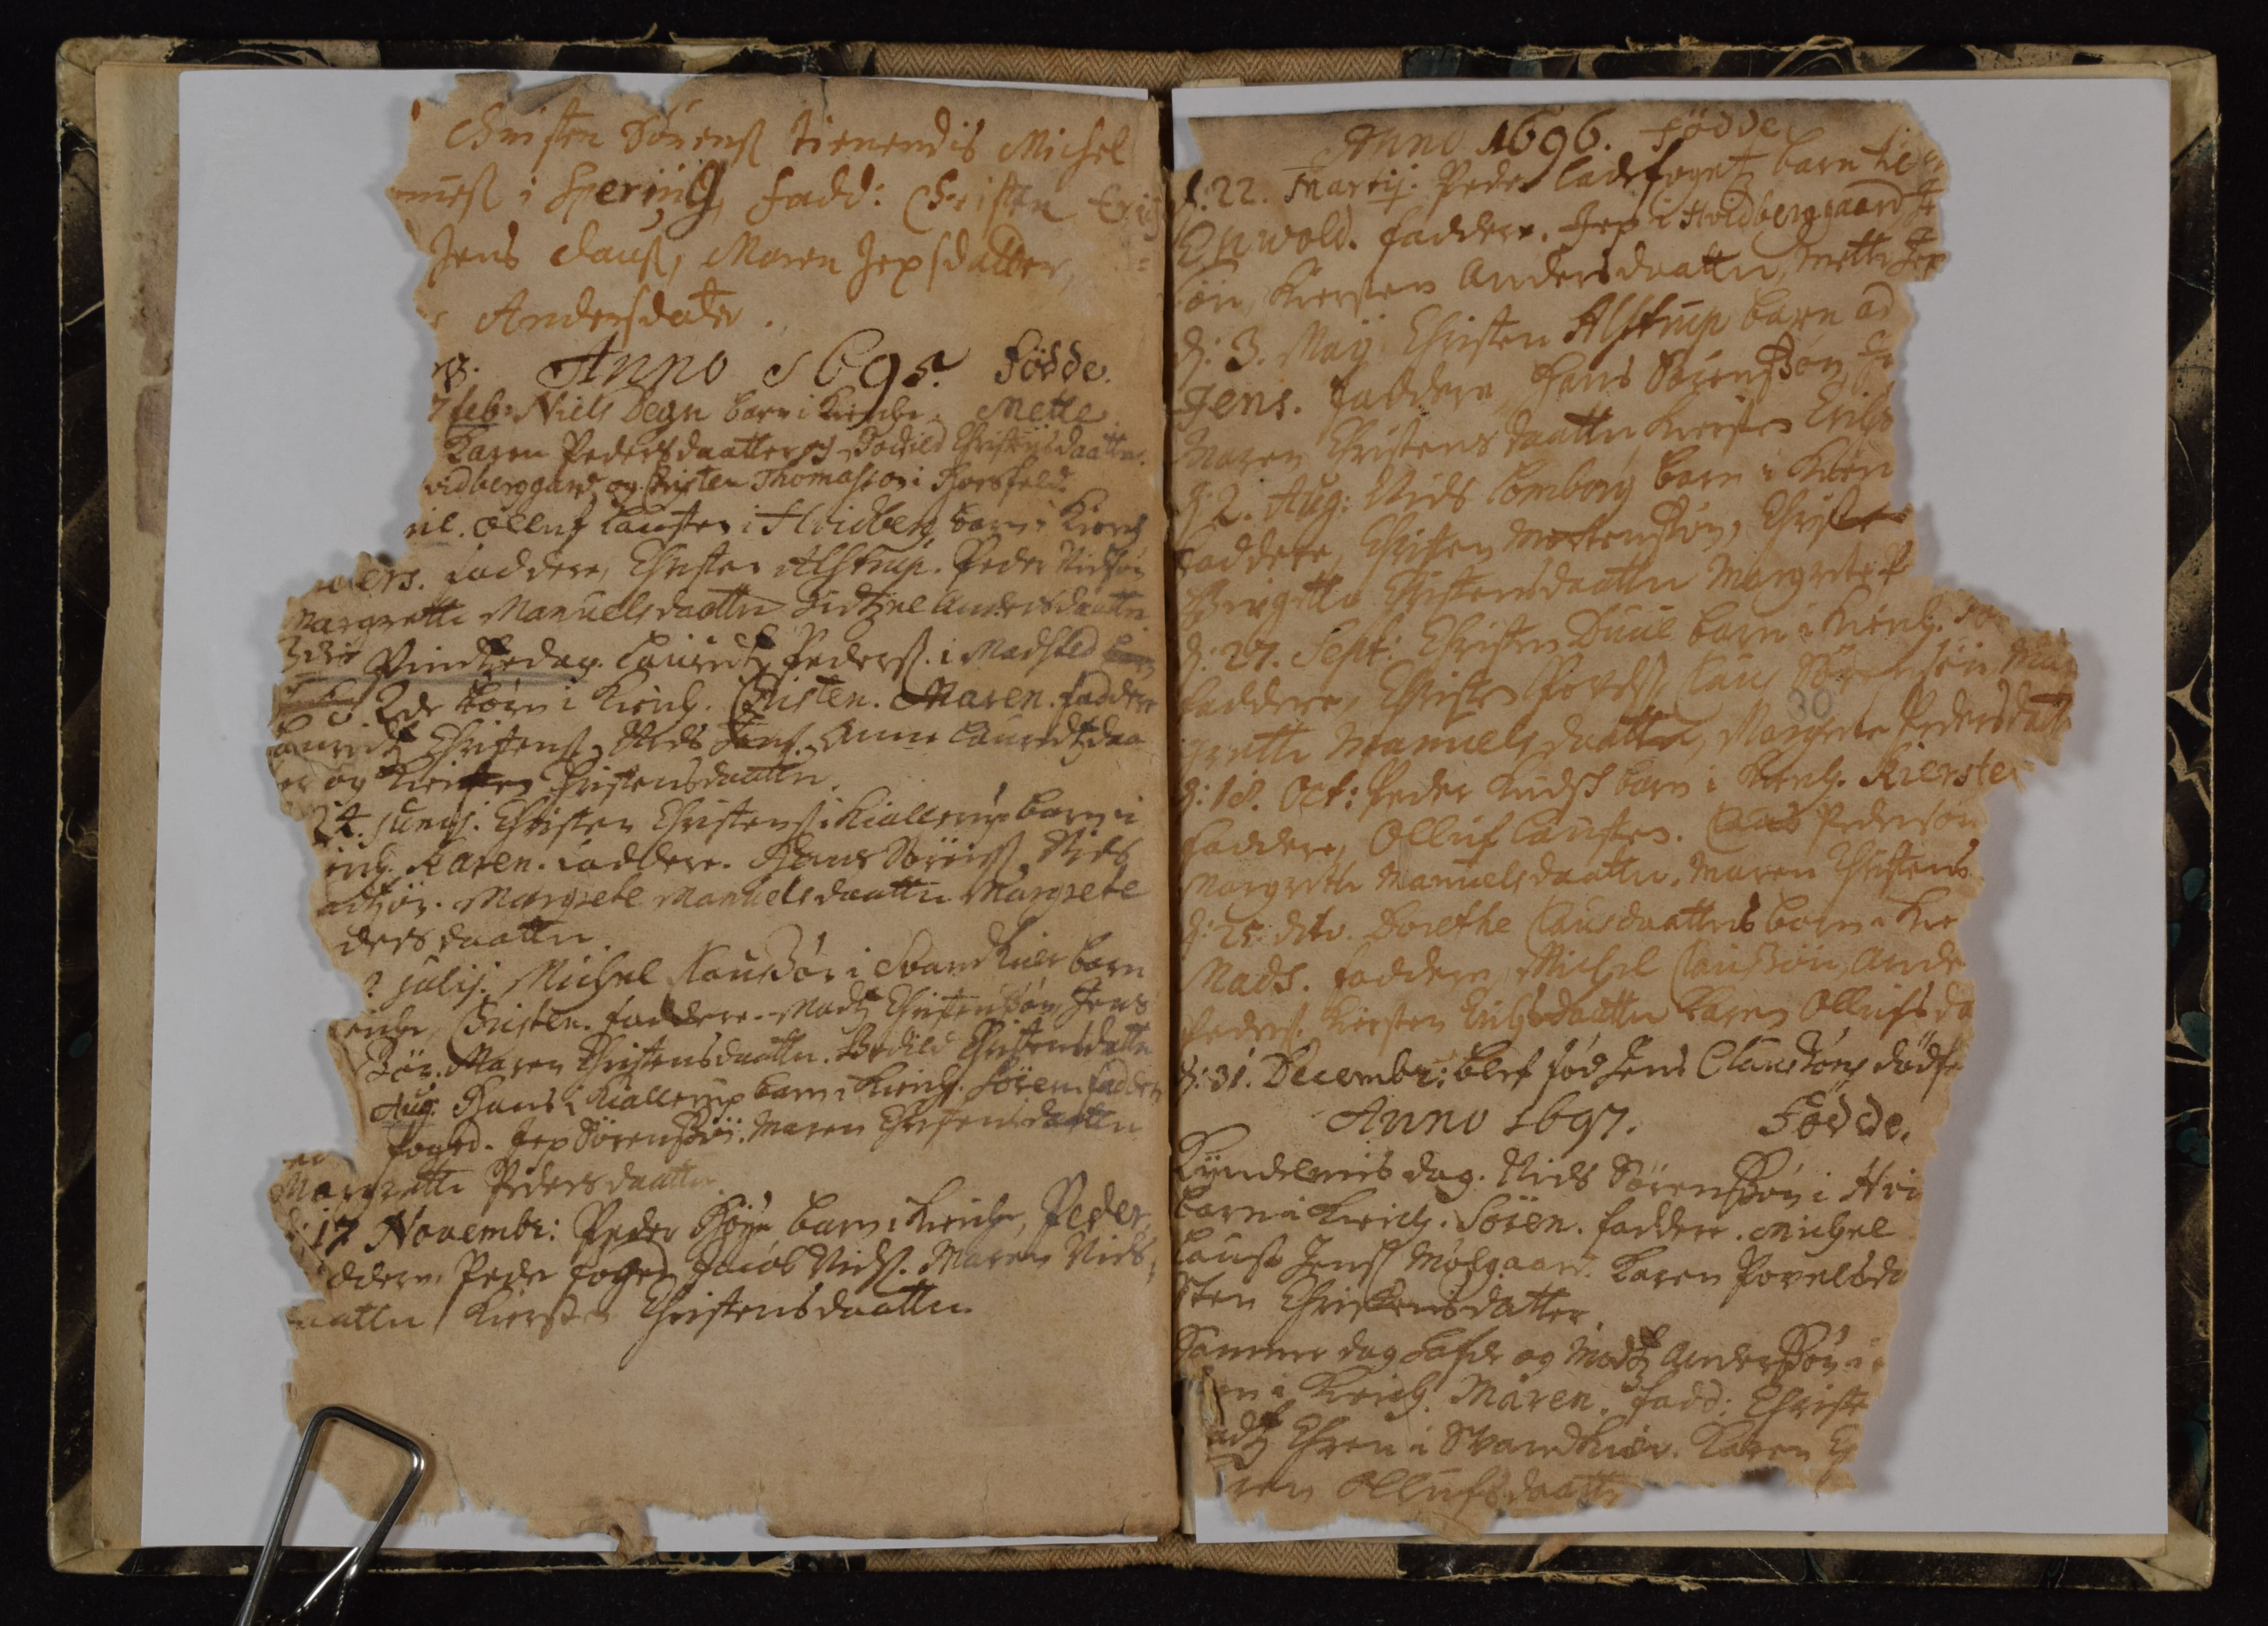Cirsten Sørens tienendis Michel
ust i Spering, Fadd: Christen Eri
Jens claus, Maren Jepsdatter
Andersdatter.
Anno 1695 Fødde
7 Feb: Niels Degn barn i Kirche Mette
Karen Pedersdaatter ## Bodild christensdaater
vidberggard og chriten Thomasøn Tjorsiheld
## Olluf Lausten i Hvidberg, barn i Kierch,
ders. Faddere, Christen Alstrup. Peder Nielsøn
Margrette Manuelsdaatter Zidtzel Andersdaatter
3die Pindtzedag Lauridtz Peders i Madsted ##
## 2de børn i Kirch Christen. Maren. Faddere
Lauridtz Christens Niels Jens Anne Lauridtzdaa
er og Kierten Christensdaatter.
24. Junij. Christen Christens i Kiallerup barn i
rche Karen. Faddere. Hans Sørens Niles
ritzøn. Margrete Manuelsdaatter Margrete
dersdaatter
2 Julij. Michel Claussøn i Svandkier barn
che, Christen Faddere. Madtz Christenssøn, Jens
##øn. Maren Christensdaatter. Bodild Christensdatter
Aug: Hans i Kiallerup barn i Kirche Søren Fadder
Foged. Jep Sørenssøn. Maren Christensdaater
Margrette Pedersdaatter
17 Nouembr: Peder Køye barn i Kirche, Peder
ddere. Peder Foged Jacob Niels. Maren Niels
aatter Kiersten Christensdaatter
Anno 1696.
Fødde
d. 22. Martij: Pedr Ladefoget barn til
Enwold. Faddere. Jep i Hvidberggaard Je
søn, Kiersten Andersdaatter, Mette Jep
d: 3. Maij Christen Alstrup barn ad  
Jens. Faddere Hans Sørenssøn, ##
Maren Christensdaatter Kiersten E##
D 2: Aug: Niels Lomborg barn i Kirc
Faddere, Christen Mortensøn, Christen
Birgete Christensdaatter Mergrete Pe
d: 27: Sept: Christen Duul barn i Kirche##
Faddere, Christen Povels ClausSørens Ni##
grette Manuels daatter, Margrete Pedersda
d: 18 Oct: Peder Kudsk barn i Kirce. Kierste
Faddere Olluf Laustens Claus Pedersøn
Margrethe Manuelsdaatter. Maren Christens
d: 25. oct: Dorethe Clausdaatters baren i Kir
Mads. Faddere Michel Clausøn Ande
Peders Kirsten ErichsdatterKaren Ollufsda
D: 31: Decembr: blef fød Jens Claussøns dødfø
Anno 1607
Fødde.
Kyndelmis dag. Niels Sørenssøn i Hvi
barn i Kirche. Søren. Faddere Michel
Laust Jens Mølgaard. Karen Povelda
sten Christensdatter.
Samme dag hafde og Madtz Anderssøn i
rn i Kirch. Maren. Fadd: Christe
adtz Chren i Svandkiær. Karen E
##en ollufsdaatter
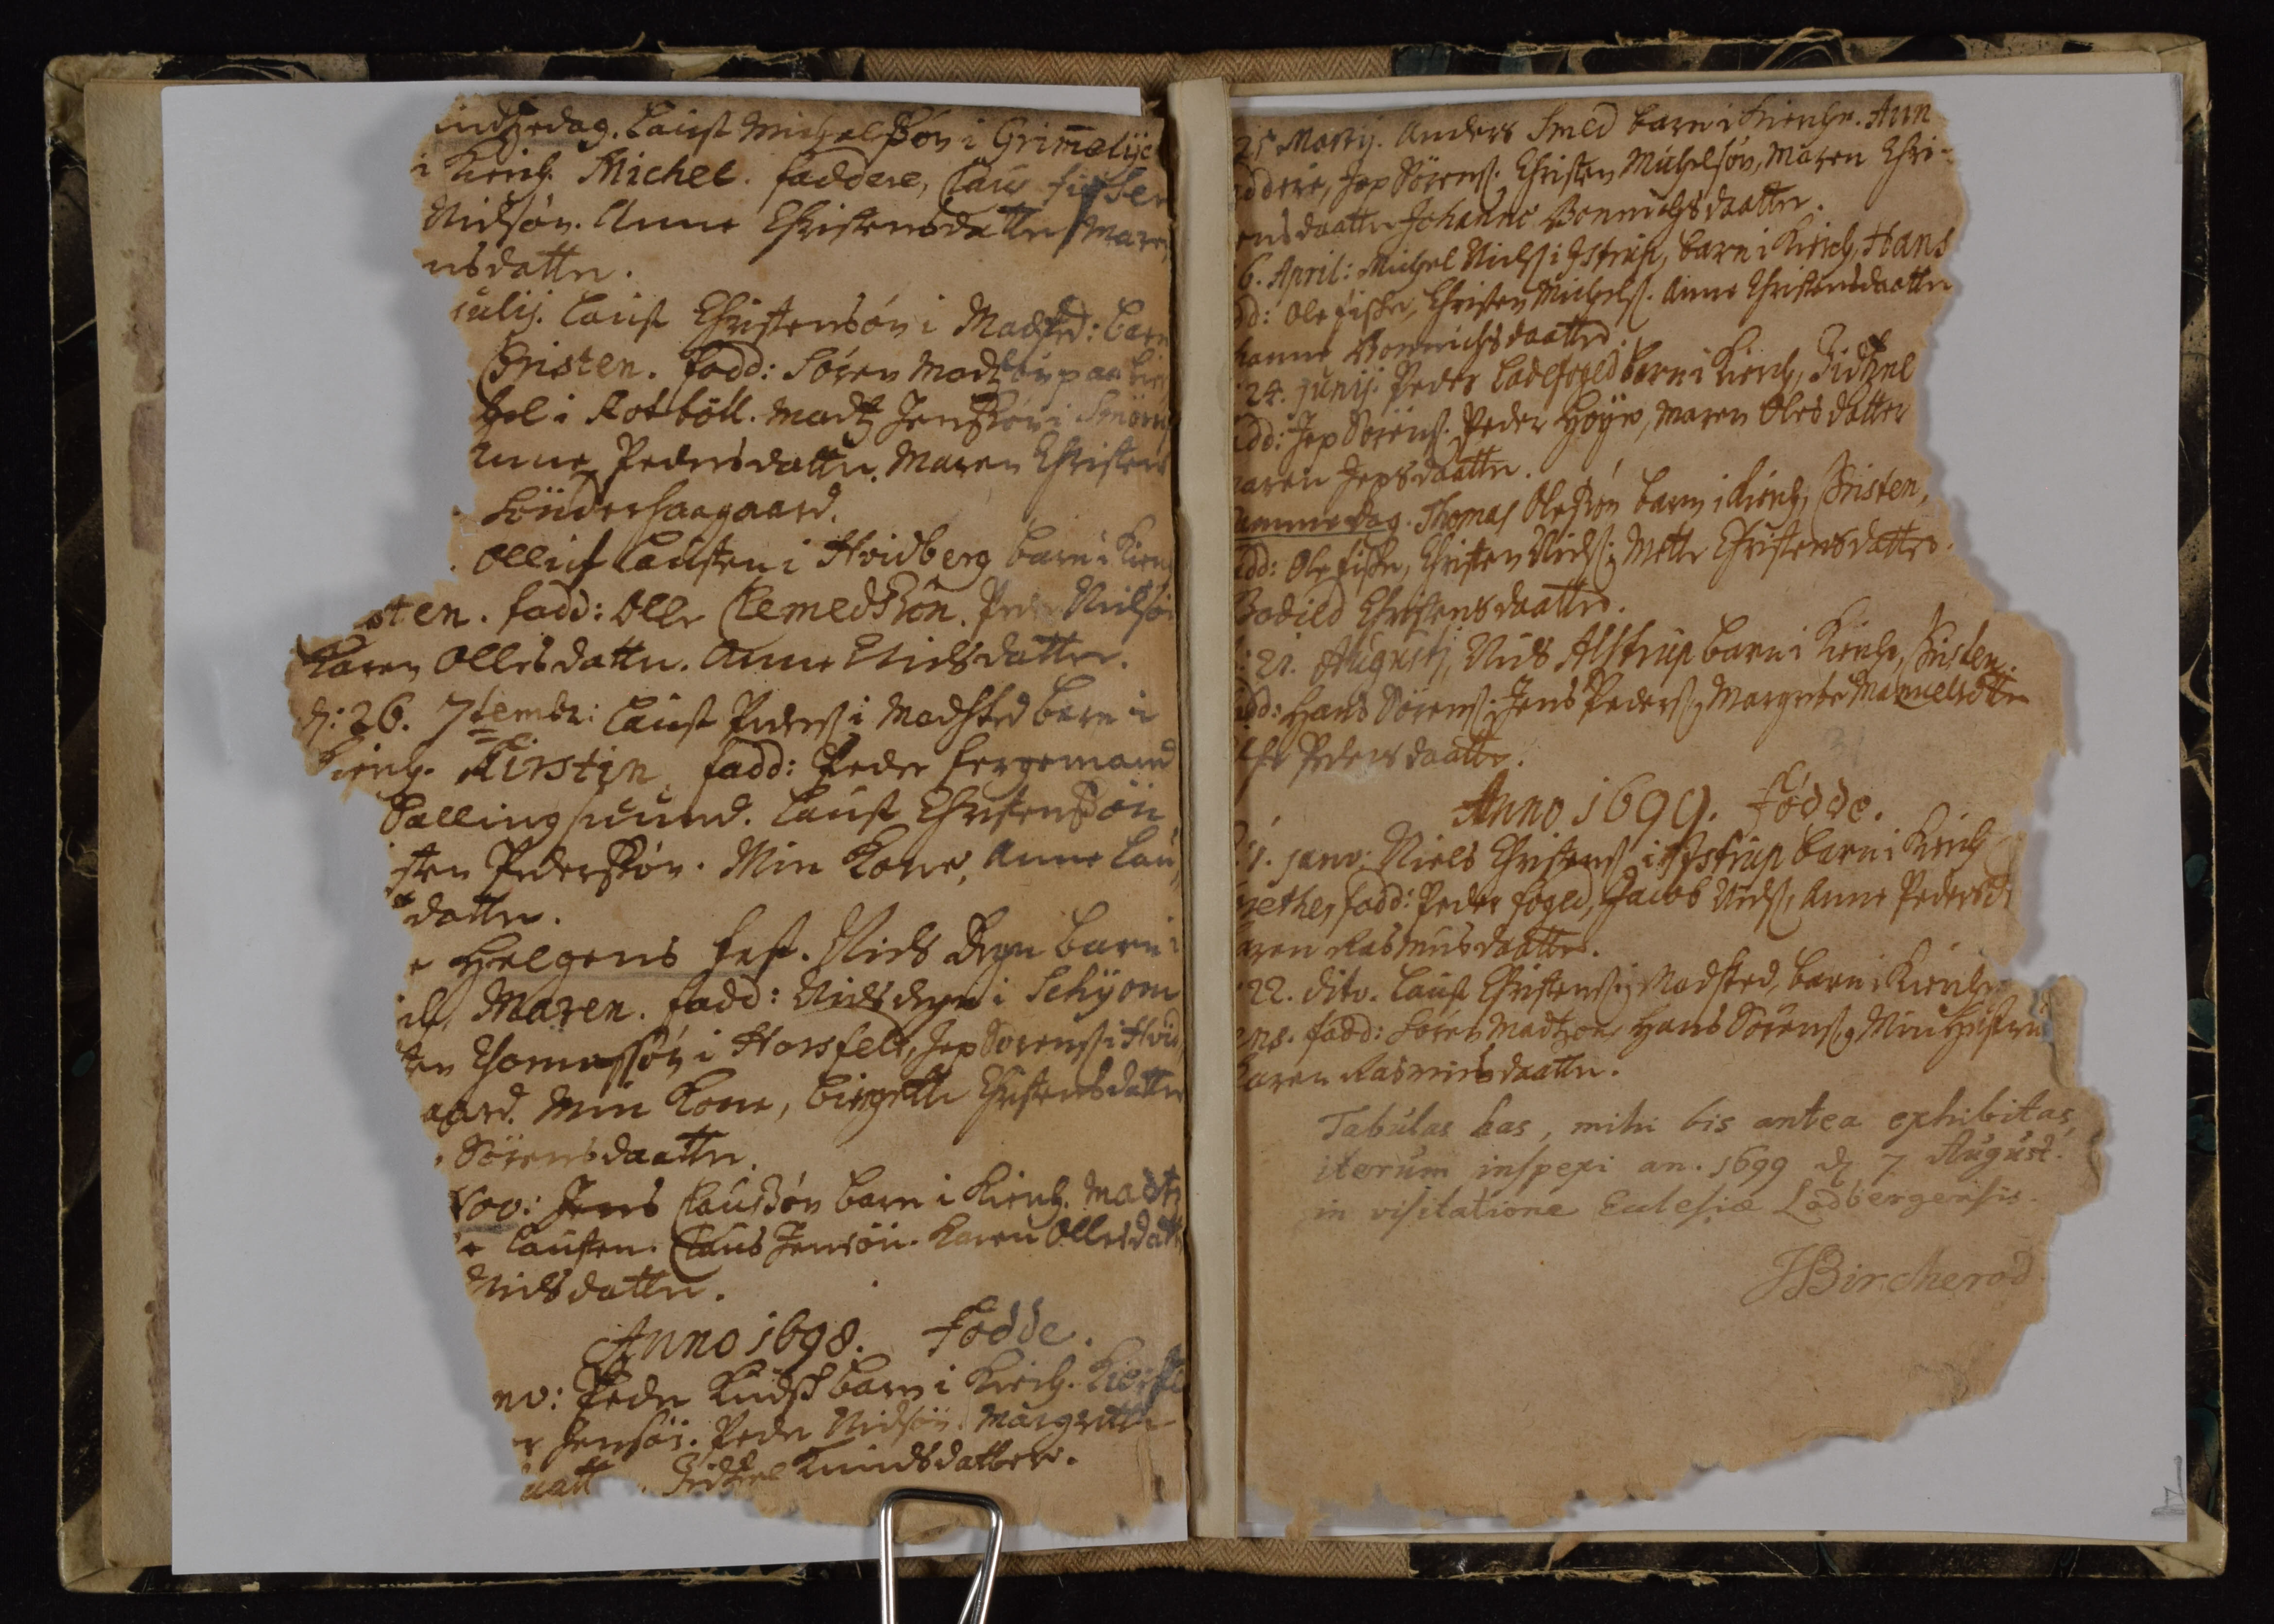indtzedag. Hans Michelssøn i Grimmolyc
i Kirch Michel. Faddere, Caus Fisher
Nielsøn. Anne Christensdatter Maren
nsdatter.
Julij. Laust Christensøn i Madsted: barn
Christen. Fadd: Søren Madtzøn paa ##
bol i Rotbøll. Madtz Jensssøn i Sønder##
Anne Pedersdatter. Maren Christens
Sønderhssgaard.
Olluf Lausten i Hvidberg barn Kir
ten. fadd: Olle Clemedssøn. Peder Nielsøn
Karen Ollesdater. Anne Nielsdatter.
d: 26. 7tembr. Laust Peders i Madsted barn i
Kirche Kirstin, fadd: Peder fergemand
Salling suund. Laust Christenssøn
sten Pederssøn. Min Kone, Anne Lau¬
datter
Helgtins fest Niels Degn barn i
## Maren. Fadd: Niels degn i Schyom
ten Thomassøn i Horsfelt, Jep Sørens i Hvi¬
aard. min Kone, Birgette Christensdatter
Sørensdaatter.
Nov: Jens Claussøn barn i Kirch Madtz
Lausten Claus Jensøn Karen Ollesdatter
Nielsdatter.
Anno 1698.
Fødde
nv: Peder Kudsk barn i Kirch Kierstin
Jensøn. Peder Nielsøn, Margrette
aatt Zidzel Knudsdatter.
25 Martij. Anders Smed barn Kierche. Ann
ddere, Jep Sørens Christen Michelsøn, Maren Chri
ensdaatter Johanne Bonnichs daatter
6. April: Michel Niels i Istrup, barn i Kirch, Hans
dd: Ole fisher. Christen Michels: Anne Christensdaatter
##anne Bonnichsdaatter
24. Junij. Peder Ladefoged barn i Kirch, Zidtzel
dd: Jep Sørens. Peder Høyn, Maren Oles datter.
aren Jepsdaatter.
mme dag. Thomas Olessøn barn i Kirch. Christen
dd: Ole fisher, Christen Nielsn, Mette Christensdatter.
Bodild Christensdaatter
21. Augustj Nis Alstrup barn i Kirche Christen
dd: Hans Sørens Jens Pedersen Margrete Manuelsdatter
ese Pedersdaatter
Anno 1699.
Fødde
1. Janv: Niels Christens i Istrup barn i Kirch
##ethe, fadd: Peder foged, Jacob Niels, Anne Pedersd.
aren Rasmusdatter.
22. dito. Laust Christens i Madsted, barn i Kierche
ns. fadd: Søren Madtzøn, Hans Sørens, Min Hustru
aren Rasmisdaatter.
Tabulas has, mitii bis antea extilit Aas
iterum inspexi an 1699 d 7 August
in Visitatione Ecclesiæ Ladbergensis
J Bircherod